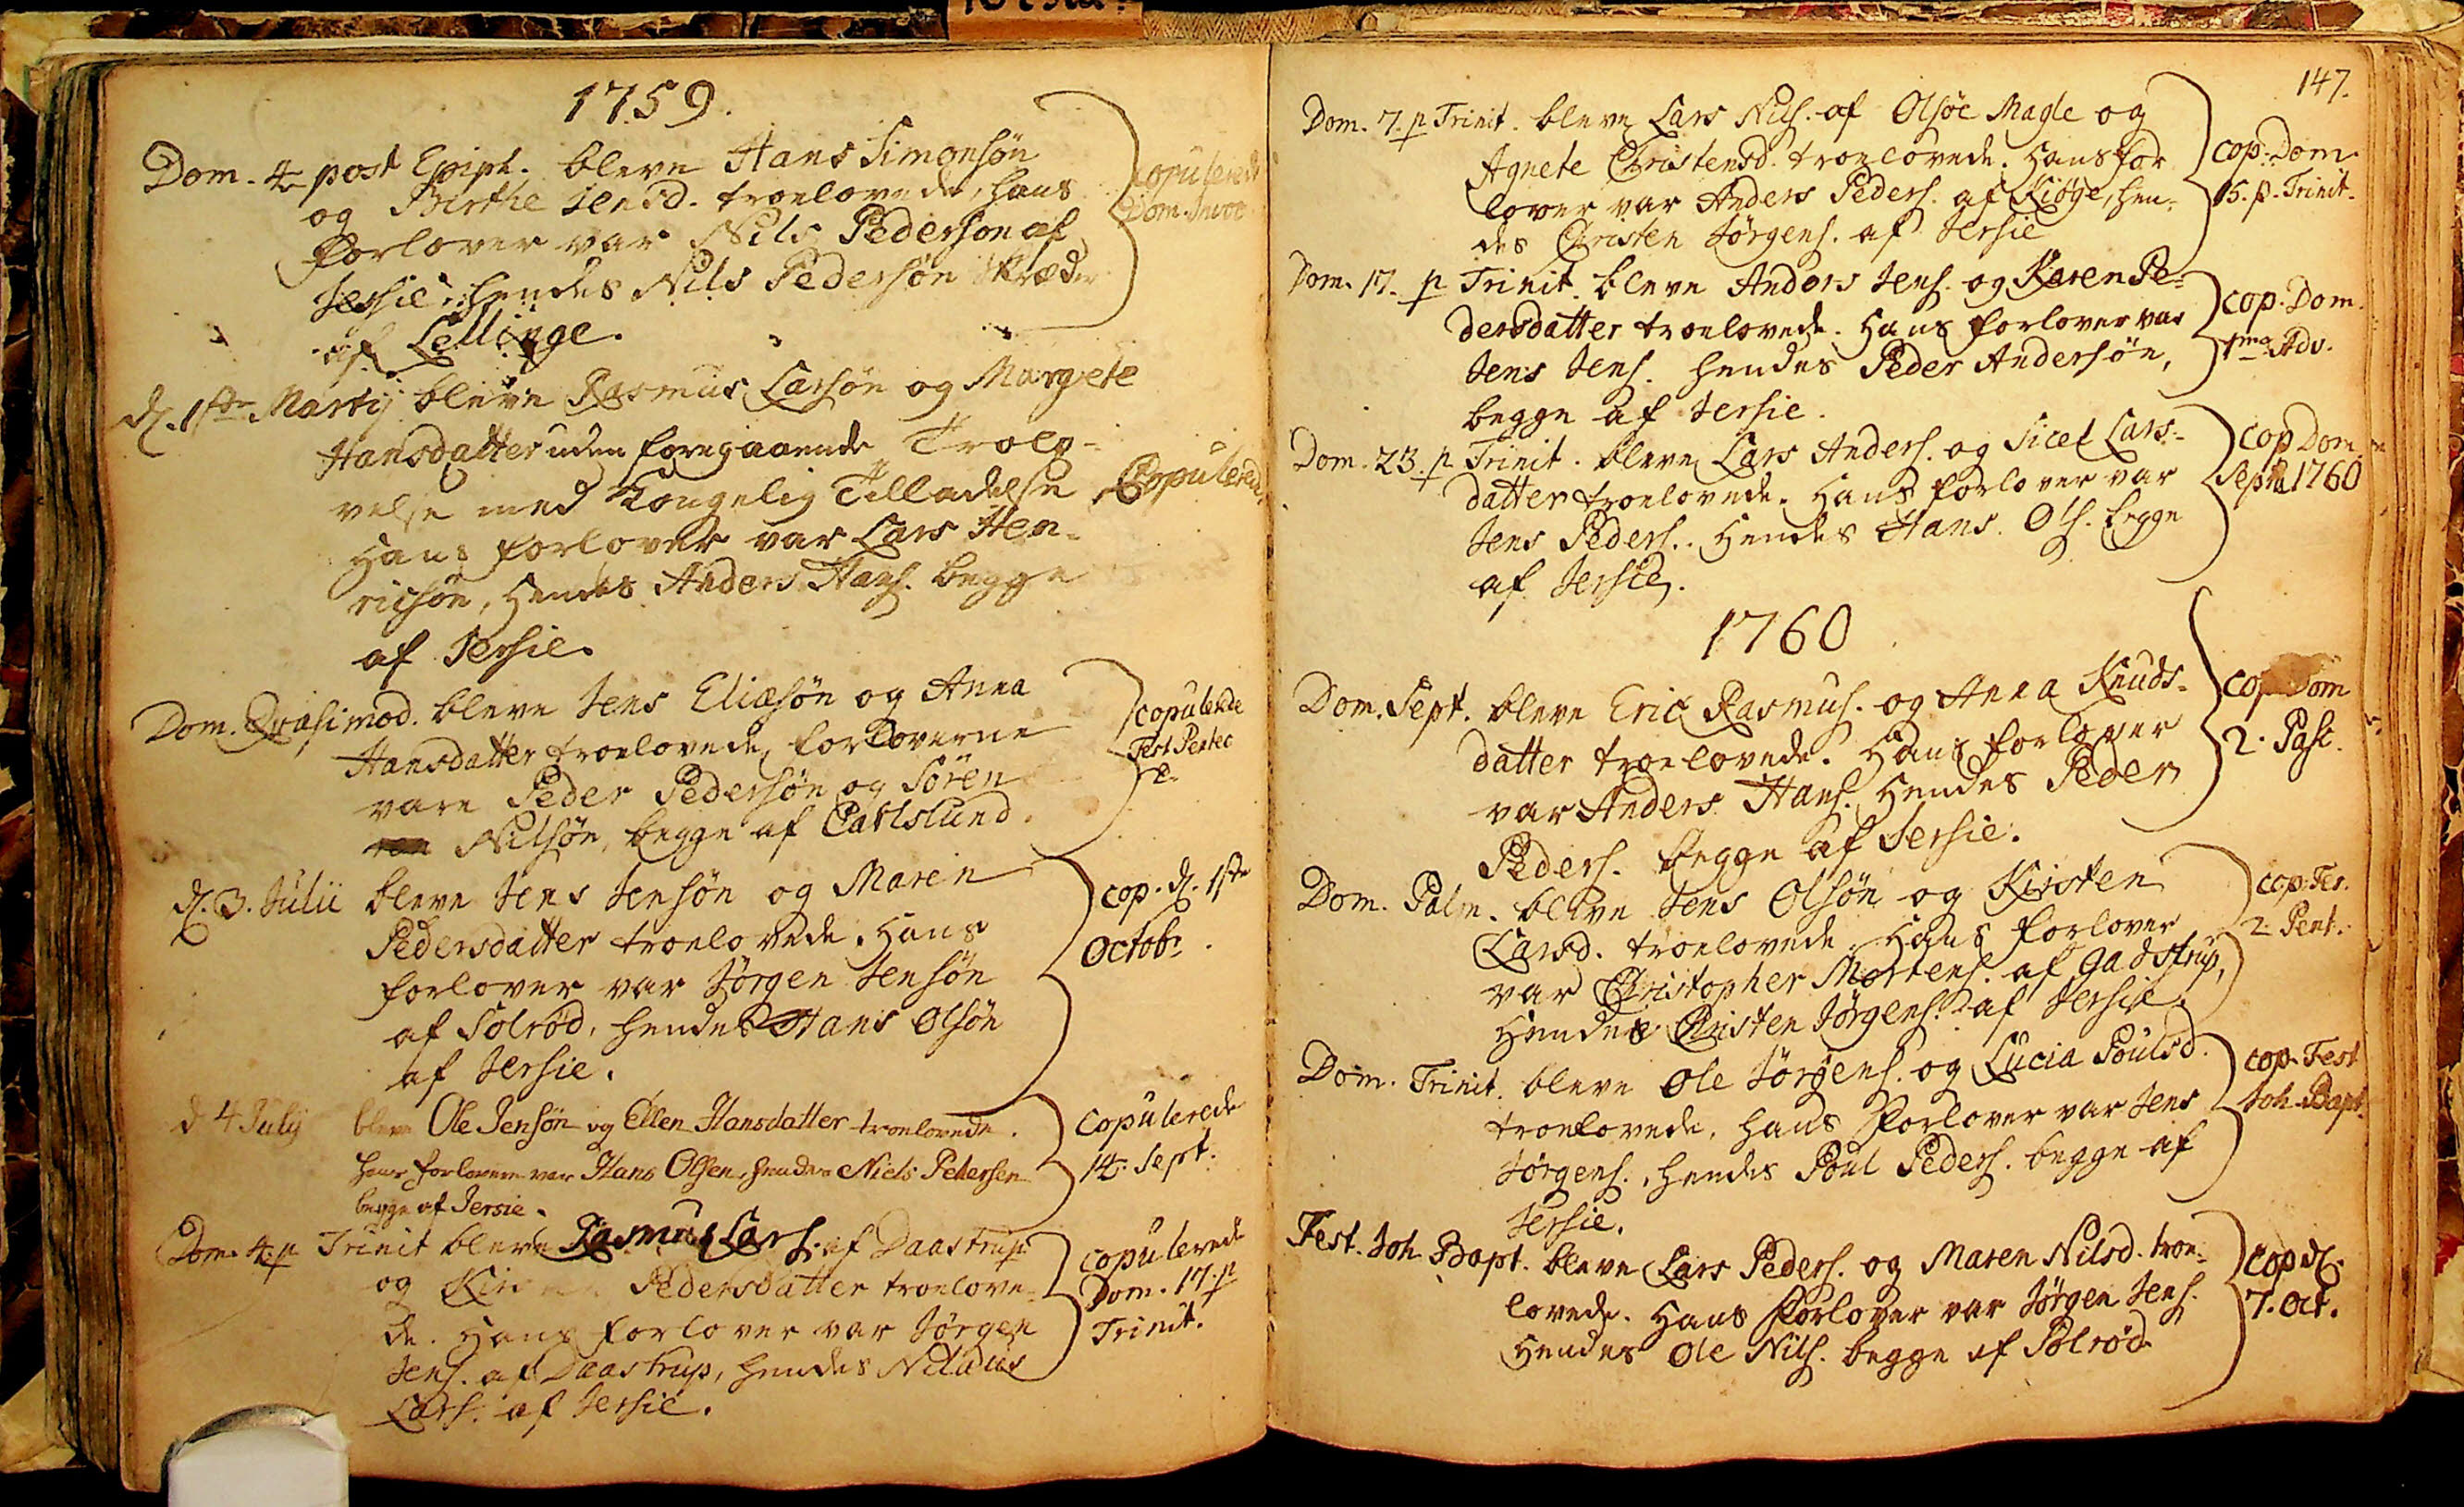1759.
Dom. 4 post Epiph. bleve Hans Simonsøn
og Birthe Jensd. troelovede, hans
forlover var Nils Pedersøn af
Jersie, hendes Nils Pedersøn Skræder
af Lellinge.
Copulerede
Dom. Invoc
d. 1ste Martij bleve Rasmus Larsøn og Margrete
Hansdatter uden foregaaende Troelo¬
velse med Kongelig Tilladelse
Hans forlover var Lars Hen¬
ricsøn, Hendes Anders Hans. begge
af Jersie.
Copulerede
Dom. Qvasimod. bleve Jens Eliæsøn og Anna
Hansdatter troelovede, forloverne
vare Peder Pedersøn og Søren
Nilsøn, begge af Carlslund.
Copulerede
Fest Pentec
##
d. 3. Julii bleve Jens Jensøn og Maren
Pedersdatter troelovede. Hans
forlover var Jørgen Jensøn
af Solrød, hendes Hans Olsøn
af Jersie.
Cob. d. 1ste
Octobr.
d 4 Julij bleve Ole Jensøn og Ellen Hansdatter troelovede.
Hans forlovere var Hans Olsen, hendes Niels Petersen
begge af Jersie.
Copulerede
14. Sept.
Dom. 4. p. Trinit bleve Rasmus Lars. af Daastrup
og Kirsten Pedersdatter troelove¬
de. Hans forlover var Jørgen
Jens. af Daastrup, hendes Nilaus
Lars. af Jersie.
Copulerede
Dom. 17. p
Trinit.
147.
Dom. 7. p Trinit. bleve Lars Niels. af Ølsøe Magle og
Agnete Christensd. troelovede. Hans for
lover var Anders Peders. af Kiøge, hen¬
des Christen Jørgens. af Jersie
Cop: Dom.
15. p. Trinit.
Dom. 17. p Trinit bleve Anders Jens. og Karen Pe¬
dersdatter troelovede. Hans forlover var
Jens Jens. hendes Peder Andersøn,
begge af Jersie.
Cop. Dom.
1ma Adv.
Dom. 23. p Trinit. bleve Lars Anders. og Sicel Lars¬
datter troelovede. Hans forlover var
Jens Peders. Hendes Hans Ols. begge
af Jersie.
Cop Dom.
Septu 1760
1760
Dom. Sept. bleve Erik Rasmus. og Anna Knuds¬
datter troelovede. Hans forlover
var Anders Hans. Hendes Peder
Peders. begge af Jersie.
Cop Dom
2. Pasc.
Dom. Palm. bleve Jens Olsøn og Kirsten
Larsd. troelovede. Hans forlover
var Christopher Mortens. af Gadstrup,
Hendes Christen Jørgens. af Jersie.
Cop: Fer.
2. Pent:
Dom. Trinit. bleve Ole Jørgens. og Lucia Poulsd.
troelovede, hans Forlover var Jens
Jørgens., hendes Poul Peders. begge af
Jersie.
Cop. Fest
Joh. Bapt.
Fest. Joh. Bapt. bleve Lars Peders. og Maren Nilsd. troe¬
lovede, Hans Forlover var Jørgen Jens.
Hendes Ole Nils. begge af Solrød. 
Cop d.
7. Oct.
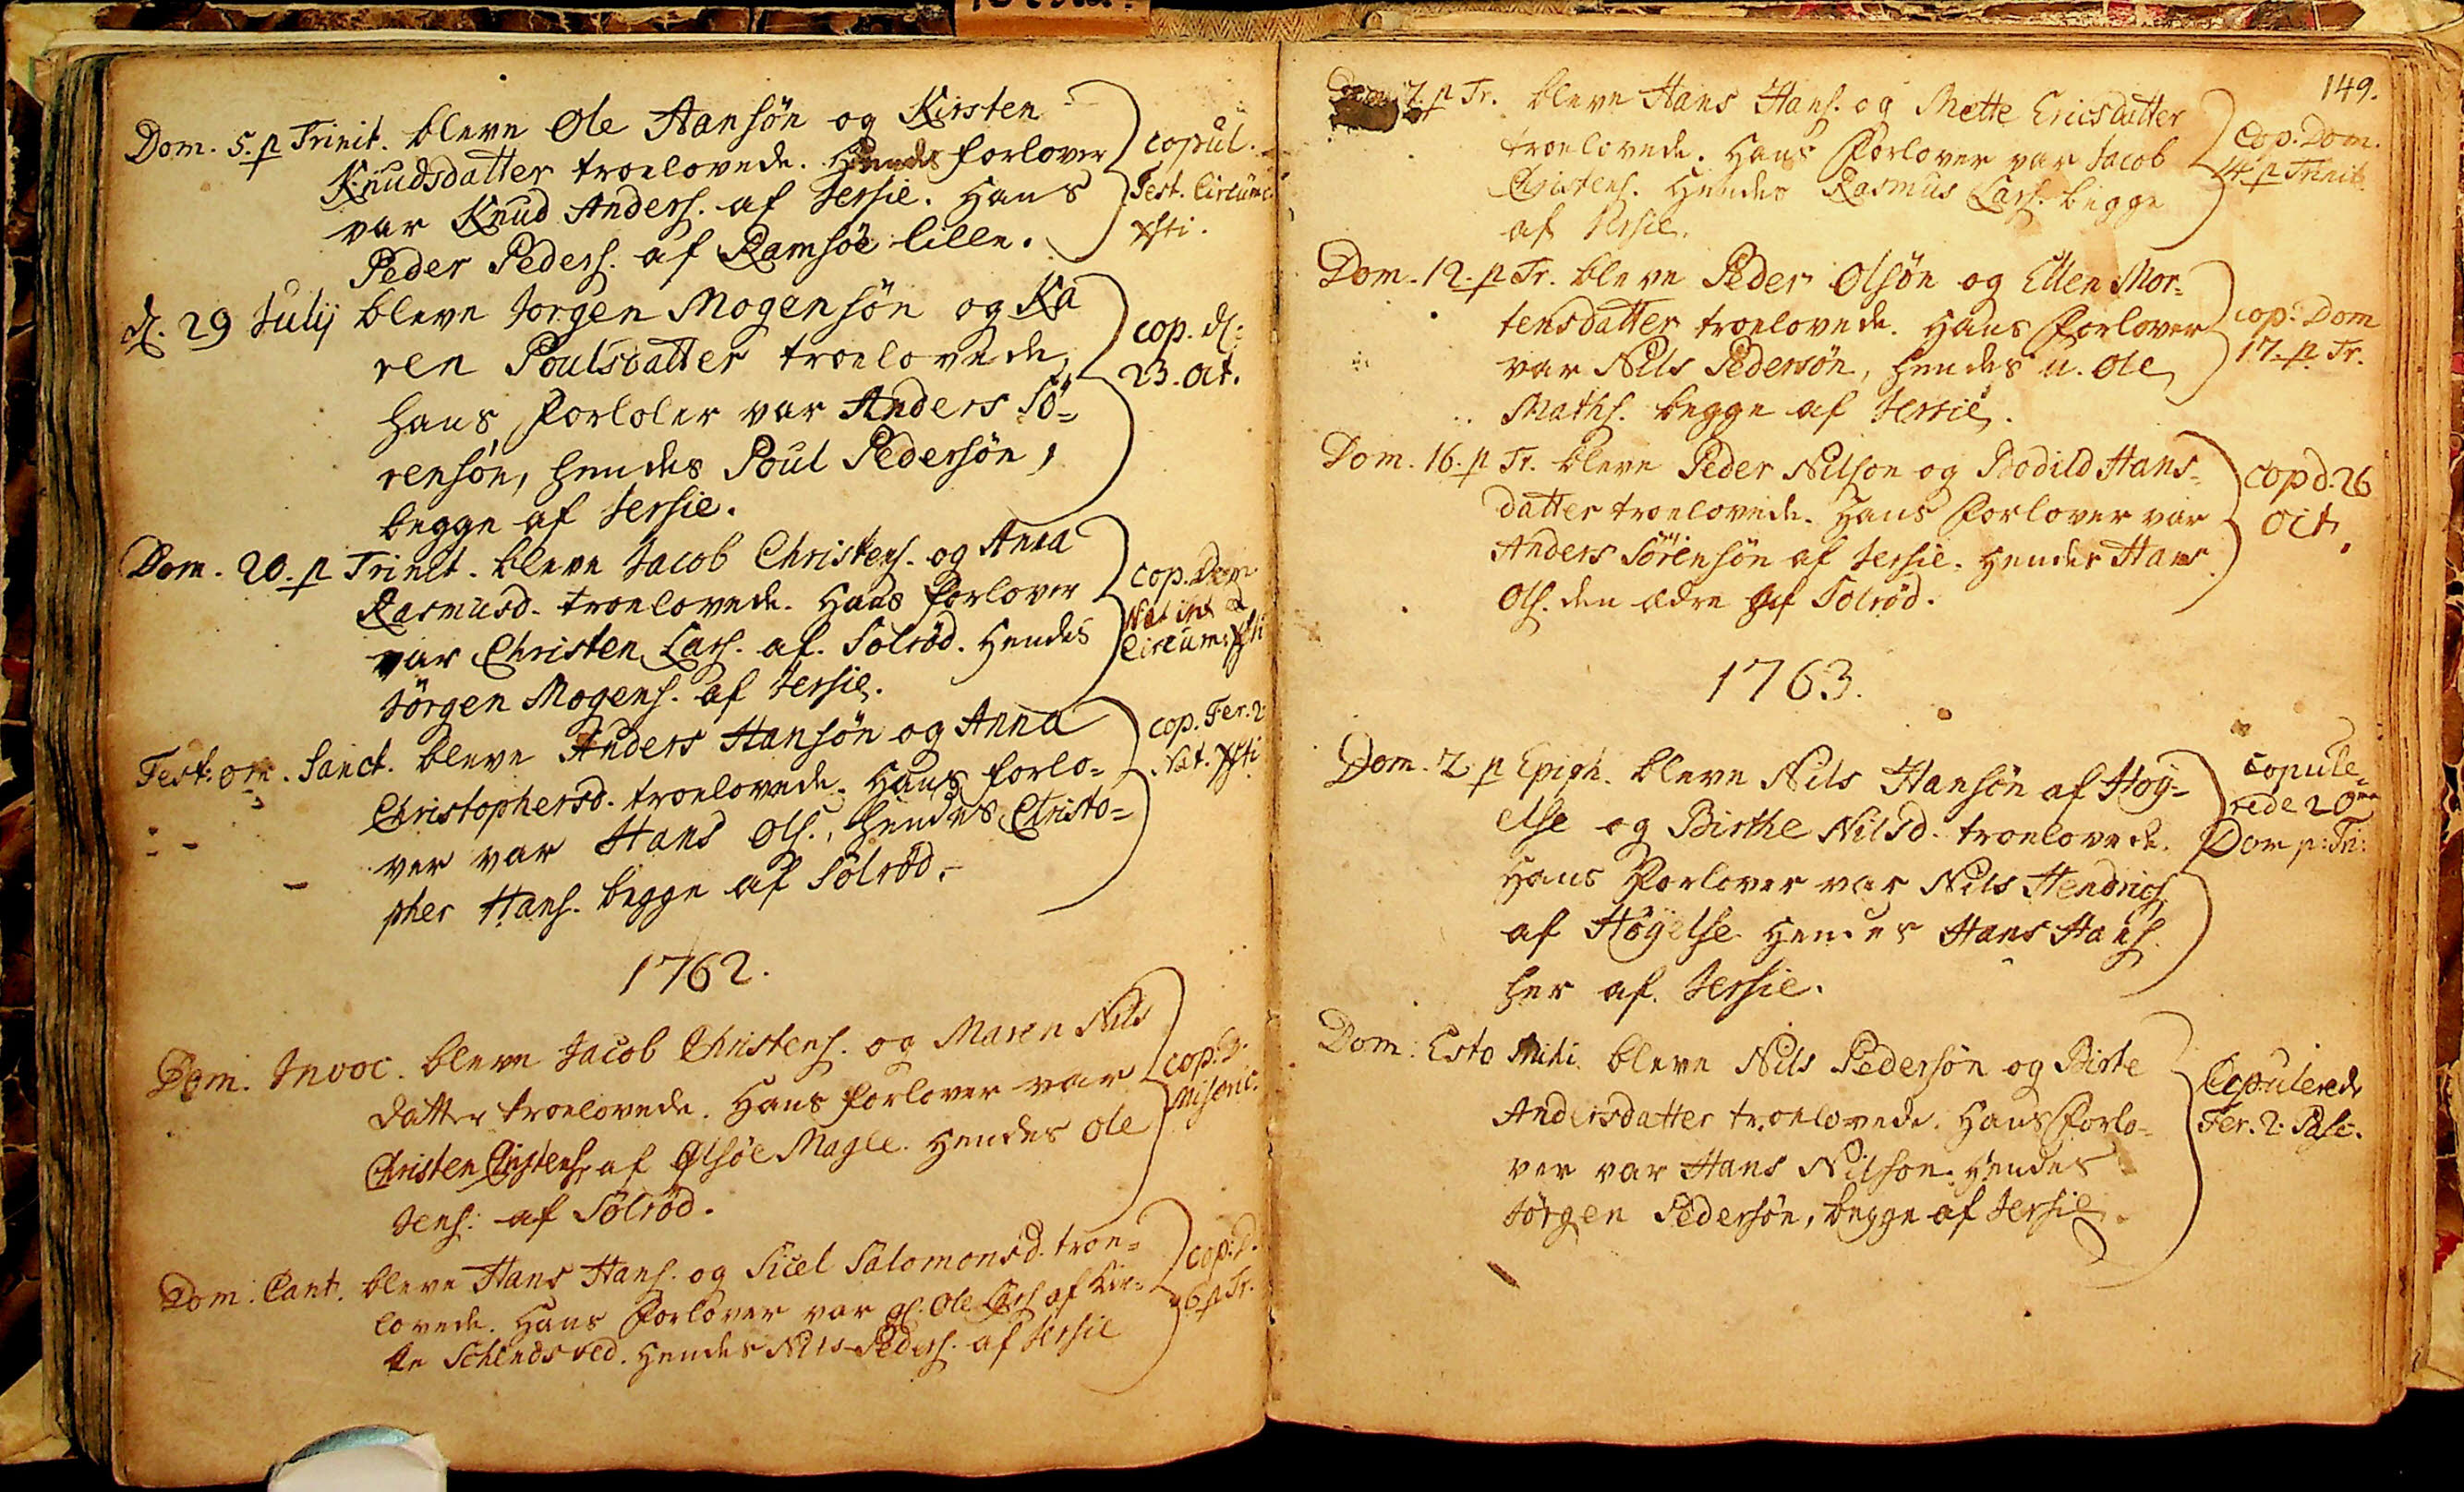Dom. 5. p Trinit. bleve Ole Hansøn og Kirsten
Knudsdatter troelovede. Hendes forlover
var Knud Anders. af Jersie. Hans
Peder Peders. af Ramsøe lille.
Copul.
Fest. Circumc
Xsti.
d. 29 Julij bleve Jørgen Mogensøn og Ka
ren Poulsdatter troelovede,
hans forlover var Anders Sø¬
rensøn, hendes Poul Pedersøn,
begge af Jersie.
Cop. d.
23. Oct.
Dom. 20. p Trinit. bleve Jacob Christens. og Anna
Rasmusd. troelovede. Hans forlover
var Christen Lars. af Solrød. Hendes
Jørgen Mogens. af Jersie.
Cop. Dom.
Nat int ex
Circum: Xsti
Fest: om. Sanct. bleve Anders Hansøn og Anna
Christophersd. troelovede. Hans forlo¬
ver var Hans Ols., hendes Christo¬
pher Hans. begge af Solrød.
Cop. Fer. 2.
Nat. Xsti
1762.
Dom. Invoc. bleve Jacob Christens. og Maren Nils
datter troelovede. Hans forlover var
Christen Christens. af Ølsøe Magle. Hendes Ole
Jens: af Solrød.
Cop. D.
Miseric.
Dom. Cant. bleve Hans Hans. og Sicel Salomonsd. troe¬
lovede. Hans Forlover var gl. Ole Lars af Kir¬
ke Schendsved. Hendes Nils Peders. af Jersie
Cop: D.
6 p Tr.
149.
Dom 7. p Tr. bleve Hans Hans. og Mette Ericsdatter
troelovede. Hans Forlover var Jacob
Christens. Hendes Rasmus Lars. begge
af Jersie.
Cop. Dom.
14 p Trinit.
Dom. 12. p Tr. bleve Peder Olsøn og Ellen Mor¬
tensdatter troelovede. Hans Forlover
var Niels Pedersøn, hendes u. Ole
Maths. begge af Jersie.
Cop. Dom
17. p Tr.
Dom. 16. p Tr. bleve Peder Nils0n og Bodild Hans¬
datter troelovede. Hans Forlover var
Anders Sørensøn af Jersie. Hendes Hans
Ols. den ældre af Solrød.
Cop d. 26
Oct.
1763.
Dom. 2 p Epiph. bleve Nils Hansøn af Høy¬
else og Birthe Nilsd. Troelovede.
Hans Forlover var Nils Hendrics.
af Høyelse Hendes Hans Hans.
her af Jersie.
Copule¬
rede 20ma
Dom p: Trin
Dom. Esto Mihi. bleve Nils Pedersøn og Birte
Andersdatter troelovede. Hans Forlo¬
ver var Hans Nilson. Hendes
Jørgen Pedersøn, begge af Jersie
Copulerede
Fer. 2. Pasc.